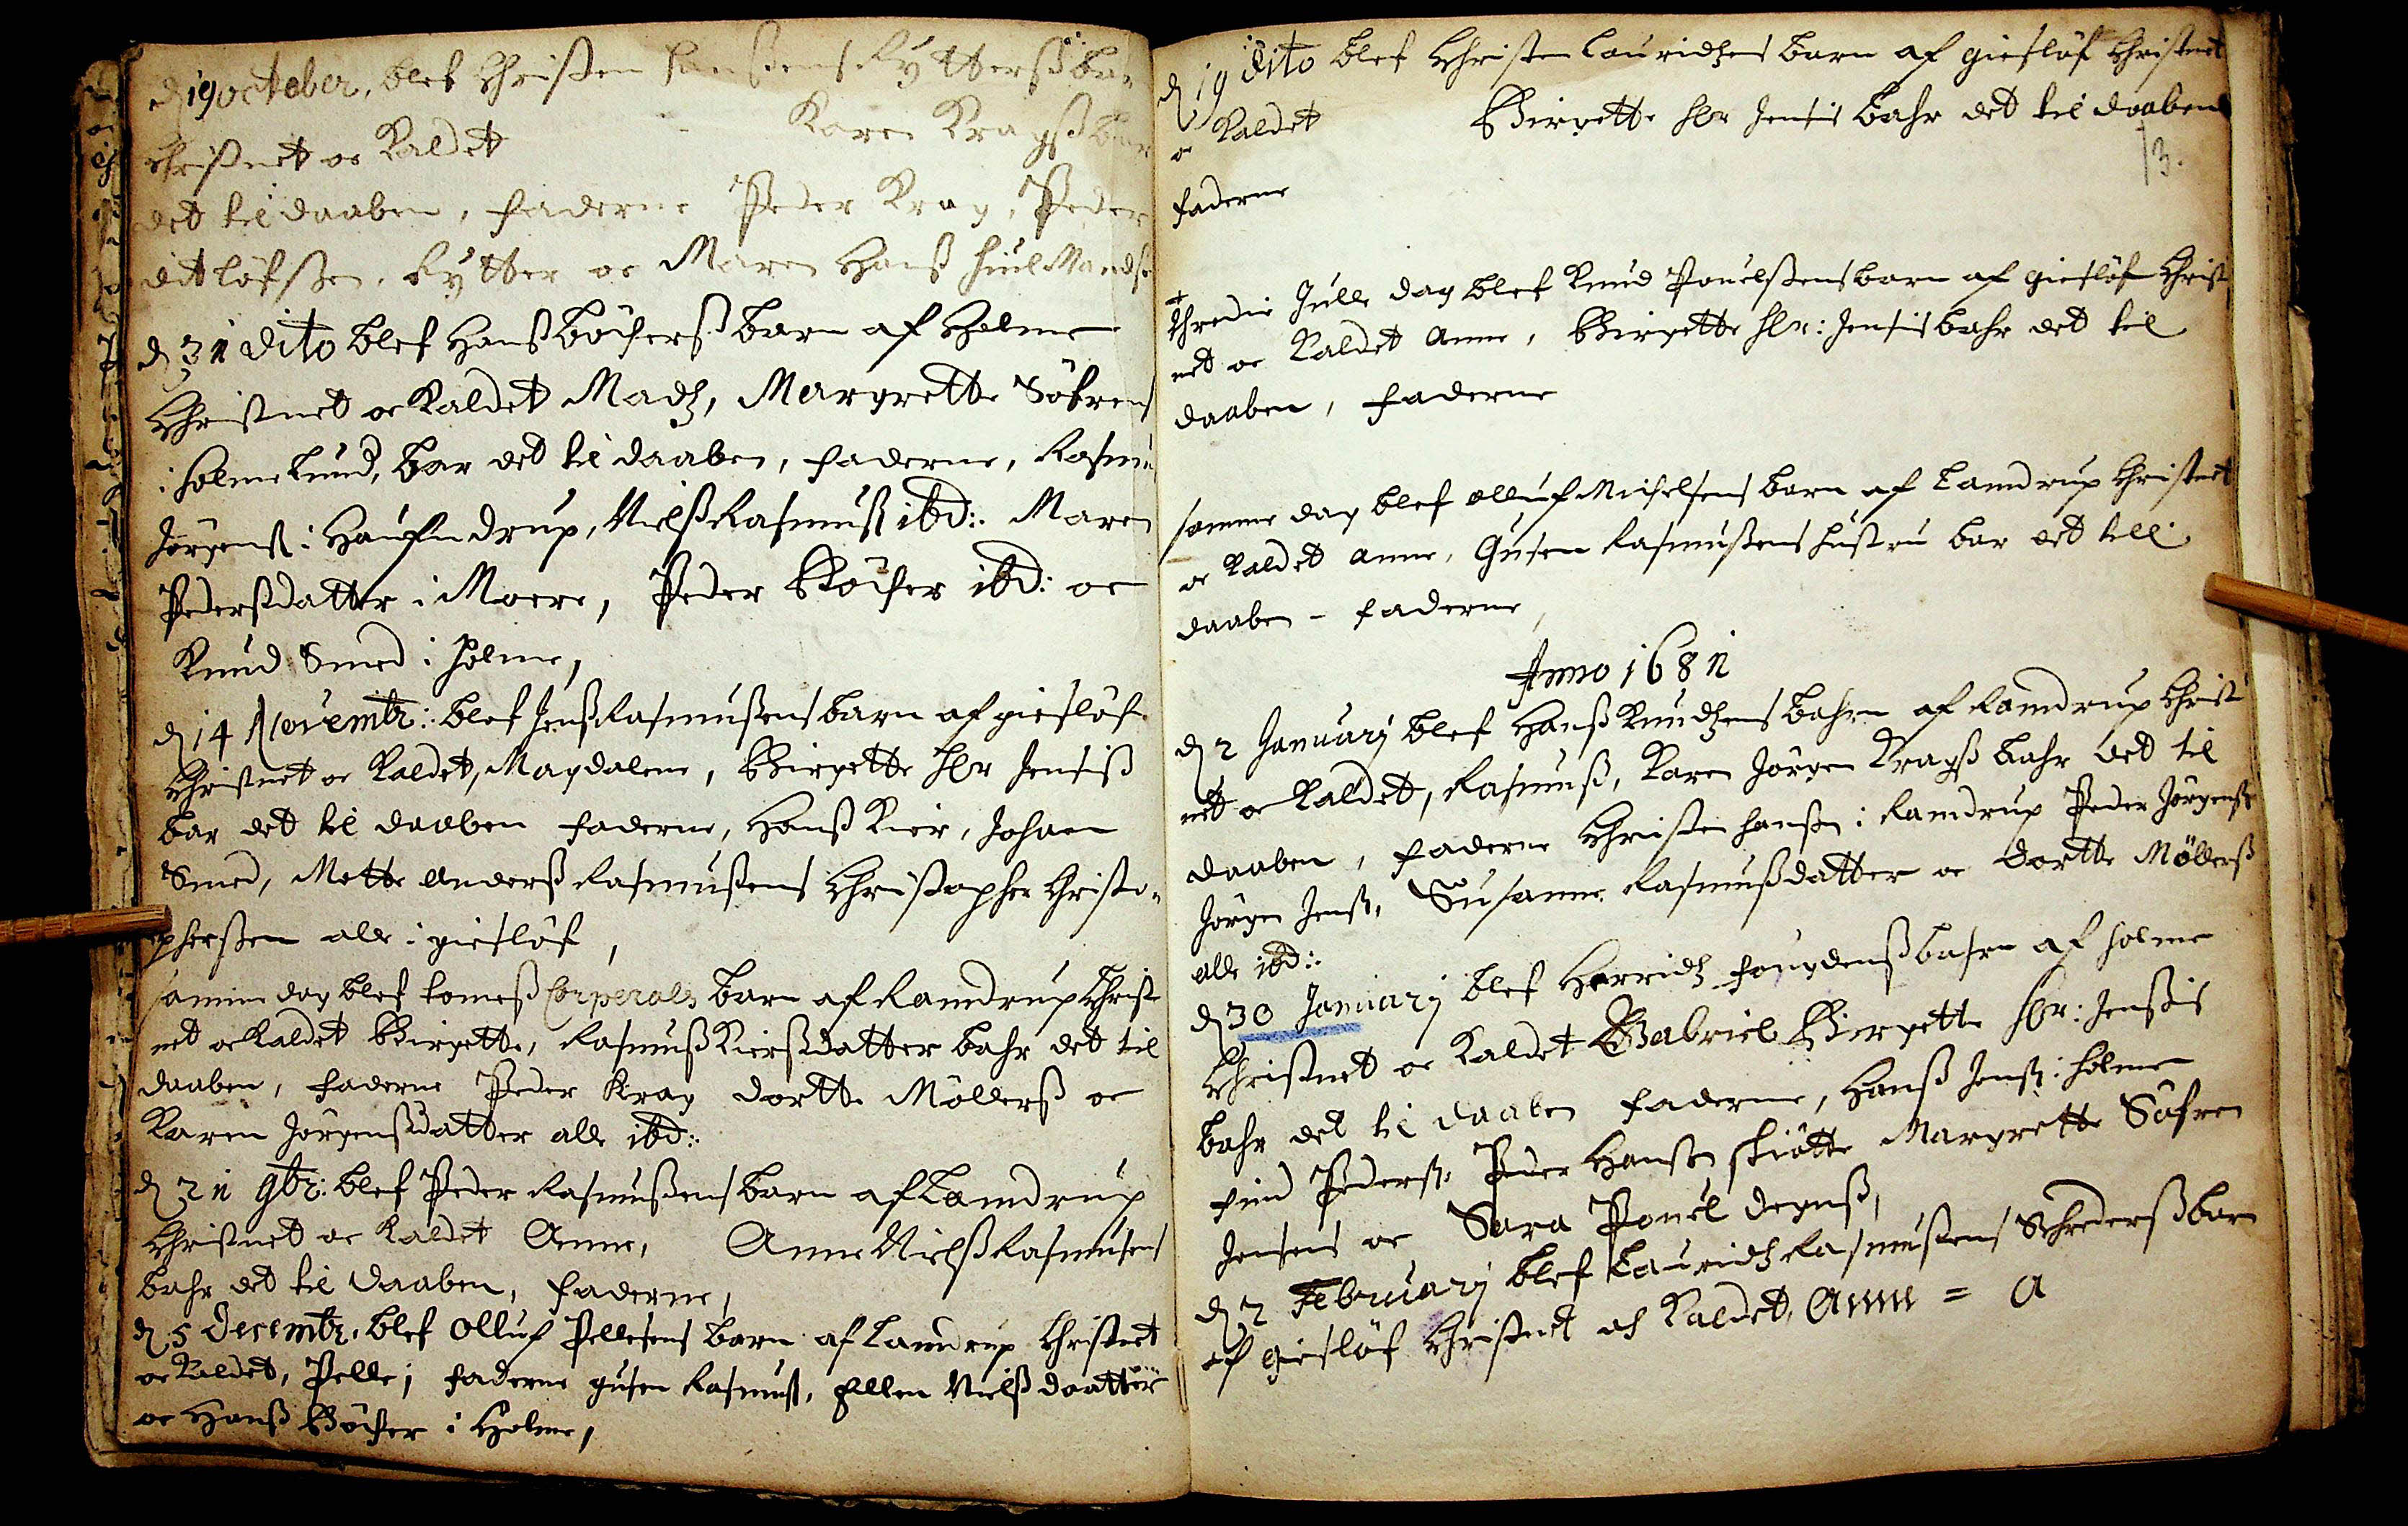d 19 october, blef Christen Hanssen Rytters bar 
Christnet oc kaldet
Karen Kragss bar
det til daaben, Faderne Peder Krag, Peder
ditløfssen Rytter oc Maren Hanss Fiul Mandsø
d 31 dito blef Hanss Bøcherss barn af Holme
Christnet oc kaldet Madtz, Margrette Søfrens
i Holmelund, bar det til daaben, Faderne, Rasmu
Jørgens i Hanufndrup, Nielss Rasmuss ibd:. Maren
Pedersdatter i Moere, Peder Bøcher ibd: oc
Knud Smed i Holme,
d 14 Novembr: blef Jenss Rasmussens barn af giesløf
Christnet oc kaldet Magdalene, Birgette hlr Jensis
bar det til daaben Faderne, Hanss Kier, Johan
Smed, Mette Anderss Rasmussens Christopher Christo¬
pherssen alle i giesløf
Samme dag blef tomess Corperols barn af Ramdrup Christ
ret oc kaldet Birgette, Rasmus Kierssdatter bahr det til
daaben, Faderne Peder Krag Dortte Mølderss oc
Karen Jørgenssdatter alle ibd:.
d 21 9br: blef Peder Rasmussens barn af Lamdrup
Christnet oc kaldet Aenne, Anne Nielss Rasmusens
bahr det til Daaben, Faderen,
d 5 Decembr. blef Olluf Pellesens barn af Lamdrup Christnet
oc kaldet, Pelle; Faderene gusen Rasmus, Ellen Nielssdaatter
oc Hanss Bøcher i Holme,
13
d 19 dito blef Christen Lauridtzens barn af giesløf Christent
oc kaldet
Birgette hlr Jensis bahr det til daaben
Faderne
Thredie Julle dag blef Knud Pouelssens barn aff Giesløf Christ
nmet oc kaldet Anne, Birgette hlr: Jensis bahr det til
daaben, Faderne
samme dag blef Olluf Michelsens barn aff Lamdrup Christenet
oc kaldet Anne, Gusen Rasmussens hustru bar det till
daaben - Faderne
Anno 1681
d 2 Januarij blef Hanss Knudtzens barrn af Ramdrup Christ
net oc kaldet, Rasmuss, Karen Jørgen Kragss bahr det til
daaben, Faderne Christen Hanssen i Ramdrup Peder Jørgens
Jørgen Jens, Susanne Rasmussdatter oc Dortte Møllerss
alle ibd:.
d 30 Januarij blef Herrodtz fougdenss af Holme
Christnet oc kaldet Gabriel Birgette hlr: Jenssis
bahr det til daaben Faderne, Hanss Jenss i Holme
Find Pederss: Peder Hanss skiøtte Margrette Søfren
Jensens oc Sara Pouel Degnss,
d 2 Februarij blef Laridtz Rasenssens Schrederss barn
af giesløf Christnet och kaldet Anne = A
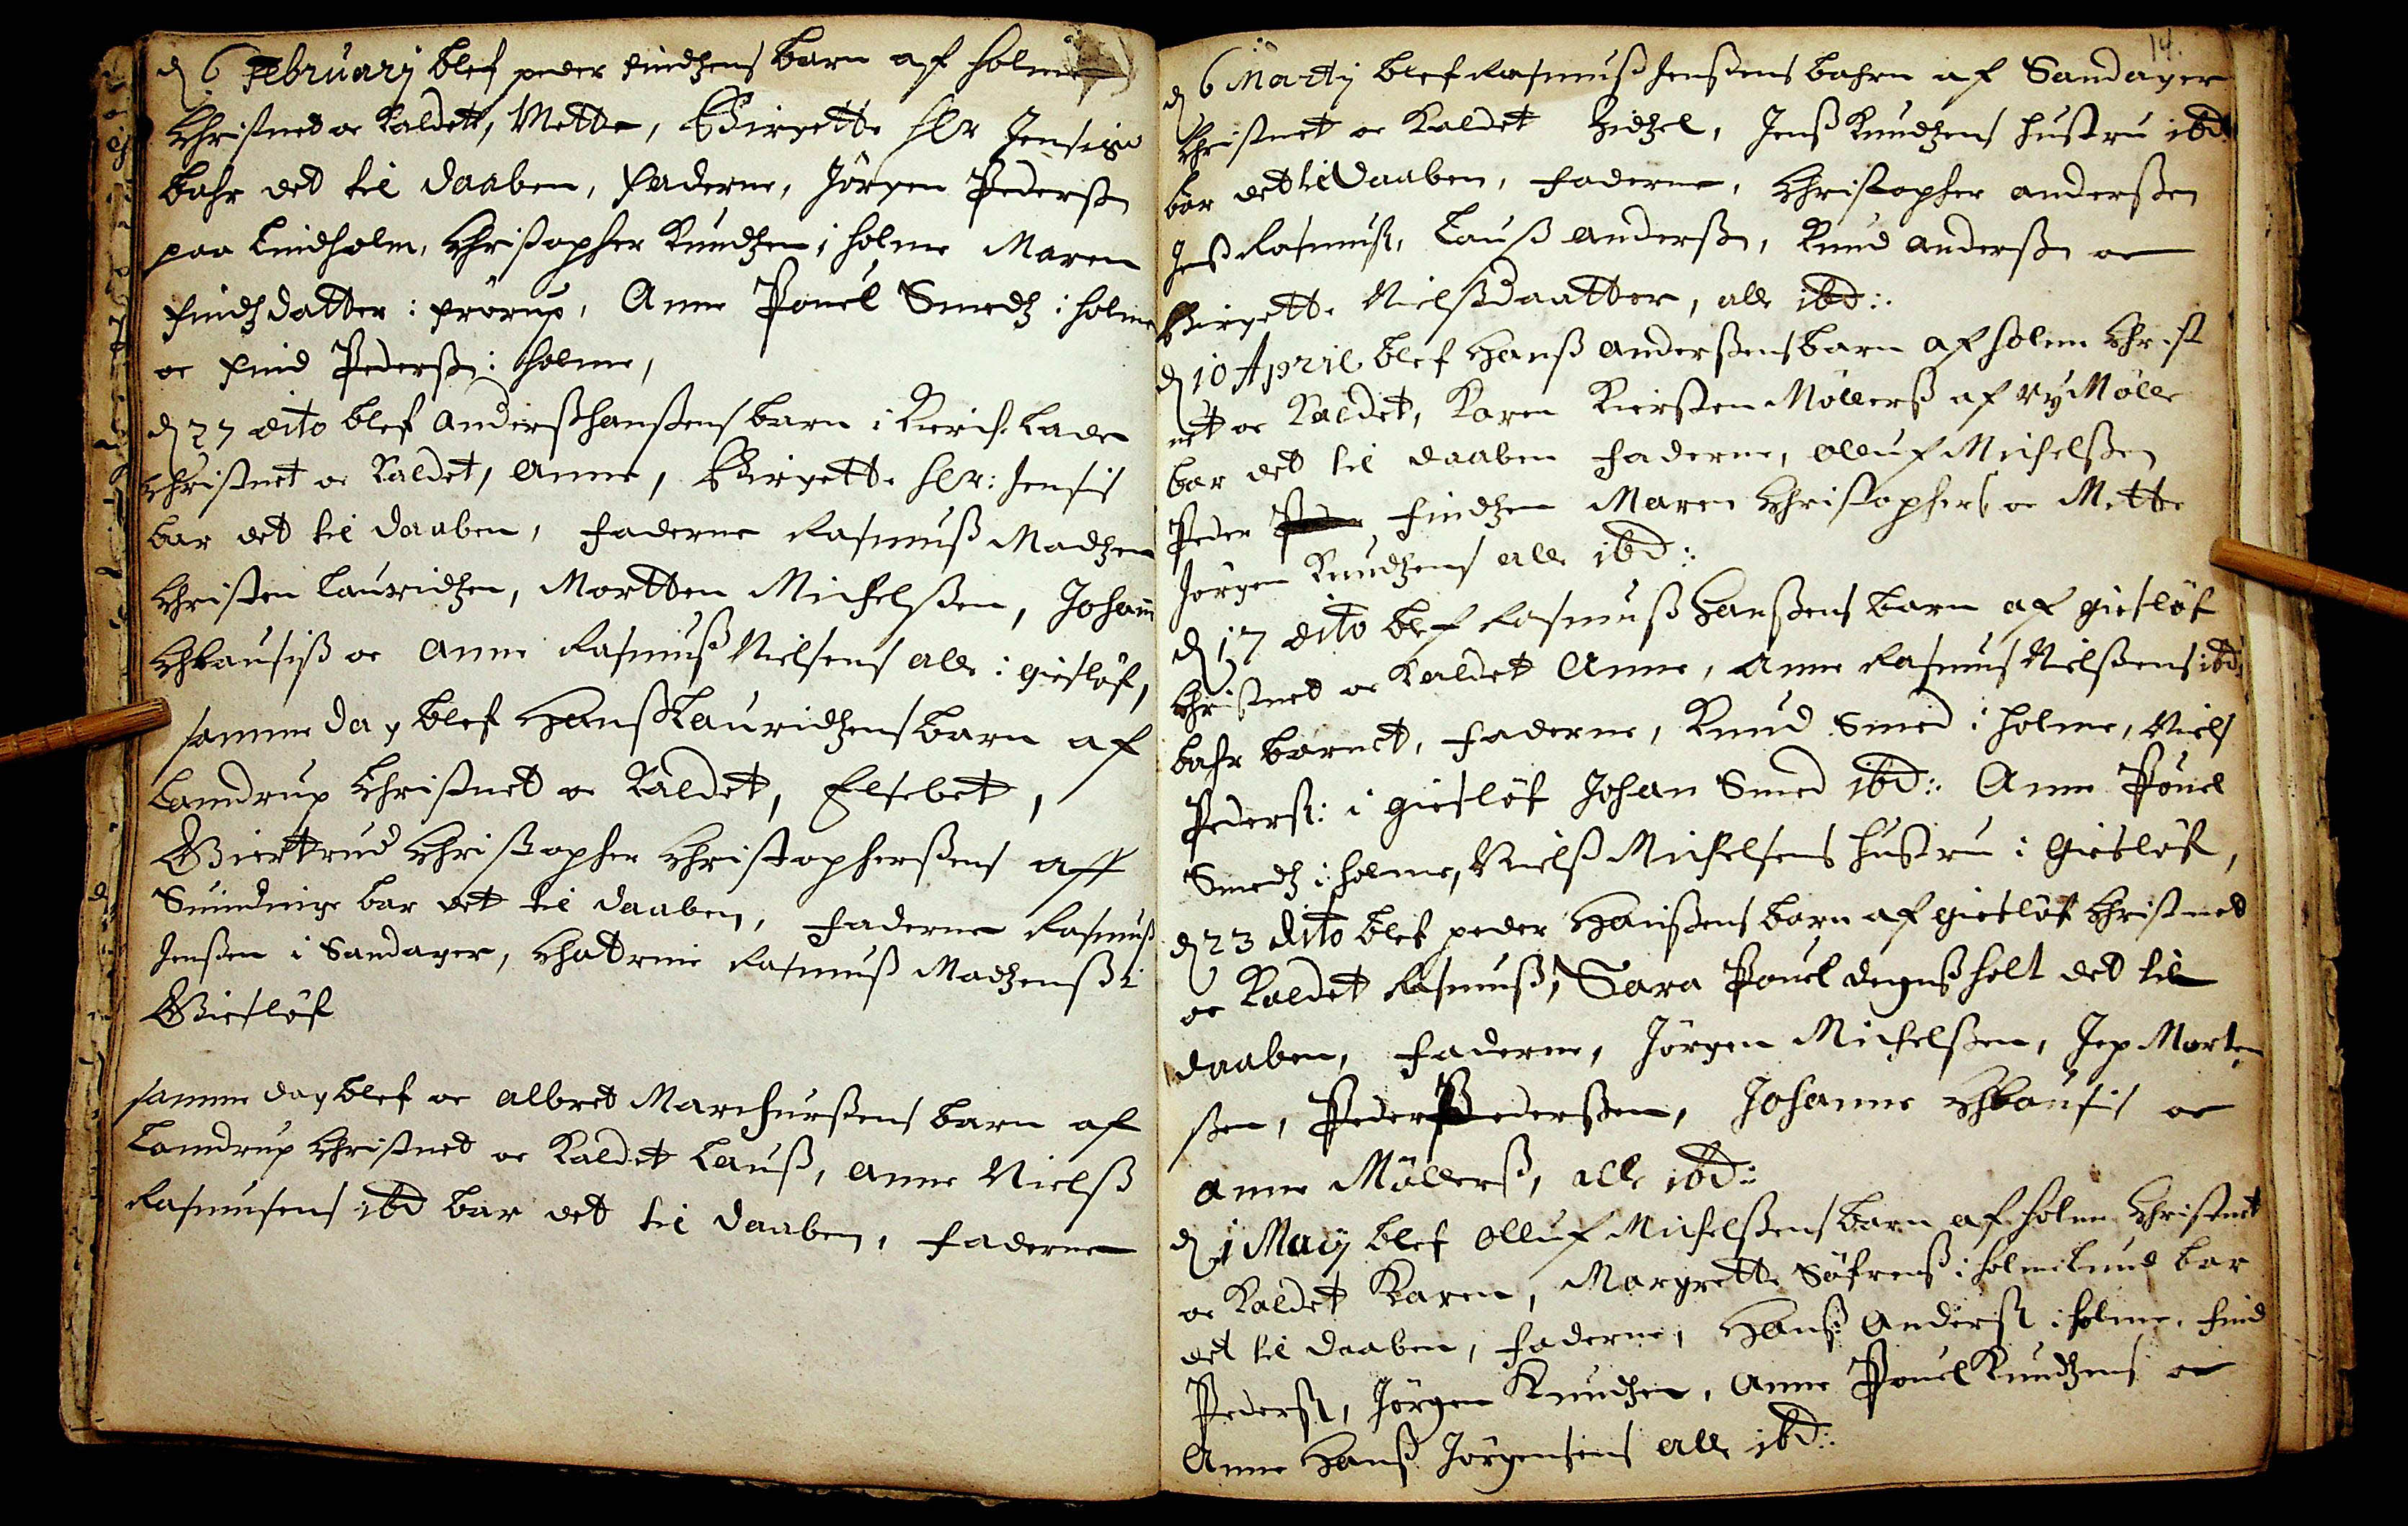d 6 Februarij blef Peder Findtzens barn af Holme
Christnet oc kaldet, Mette, Birgette hlr Jensis
bahr det til daaben, Faderne, Jørgen Pederssen
paa Lindholm, Christopher Knudtzen i holme Maren
Findtzdatter i Frørup, Anne Pouel Smeds i Holme
oc Find Pederssen i Holme,
d 27 dito blef Anderss Hanssens barn i Kierch Lade
Christnet oc kaldet, Anne, Birgette hlr: Jensis
bar det til daaben, Faderne Rasmuss Madtzen
Christen Lauridtzen, Mortten Michelssen, Johan
Chlausiss oc Anne Rasmuss Nielsens alle i Giesløf,
samme dag blef Hans Lauridtzens barn af
Lamdrup Christned oc kaldet Elsebet,
Giertrud Christopher Christopherssens aff
Suindinge bar det til daaben, Faderne Rasmuss
Jenssen i Sandager, Chatrine Rasmuss Madtzenss i
Giesløf
samme dag blef oc albret Marchurssens barn af
Landrup Christnet oc kaldet Lauss, Anne Nielss
Rasmusens ibd bar det til daaben, Faderne
14.
d 6 Martij blef Rasmuss Jenssens barrn af Sandager
Christnet oc kaldet Zidtzel, Jens Knudtzens hustrue ibd
bar det til daaben, Faderne, Christopher Anderssen
Jenss Rasmuss, Lauss Anderssen, Knud Anderssen oc
Birgette Nielssdaatter, alle ibd:.
d 10 Apriil blef Hanss Anderssens barn af holme Christ
net oc kaldet, Karen Kiersten Møllerss af ry Mølle
bar det til daaben Faderne, Olluf Michelssen
Peder P## Findtzen Maren Christophers oc Mette
Jørgen Knudtzens alle ibd:.
d 17 dito blef Rasmuss Hanssens barn af giesløf
Christenet oc kaldet Anne, Anne Rasmus Nielssens ibid
bahr barnet, Faderne, Knud Smed i holme, Niels
Pederss: i Giesløf Johan Smed ibd:. Anne Pouel
Smedtz i holme, Nielss Michelsens hustru i Giesløf,
d 23 dito blef peder Hanssens barn af Giesløf Christnet
oc kaldet Rasmuss, Sara Pouel Degnss holt det til
daaben, Faderene, Jørgen Michelssen, Jep Morten
ssen, Peder Pederssen, Johanne Chlausis oc
Anne Møllerss, alle ibd:.
d 1 Maij blef Olluf Michelssens barn af holme Christenet
oc kaldet Karen, Margrette Søfrenss i Holmelund bar
det til daaben, Faderne, Hanss Anderss i Holme, Find
Pederss, Jørgen Knudtzen, Anne Pouel Knudtzens oc
Anne Hanss Jørgensens alle ibd:.